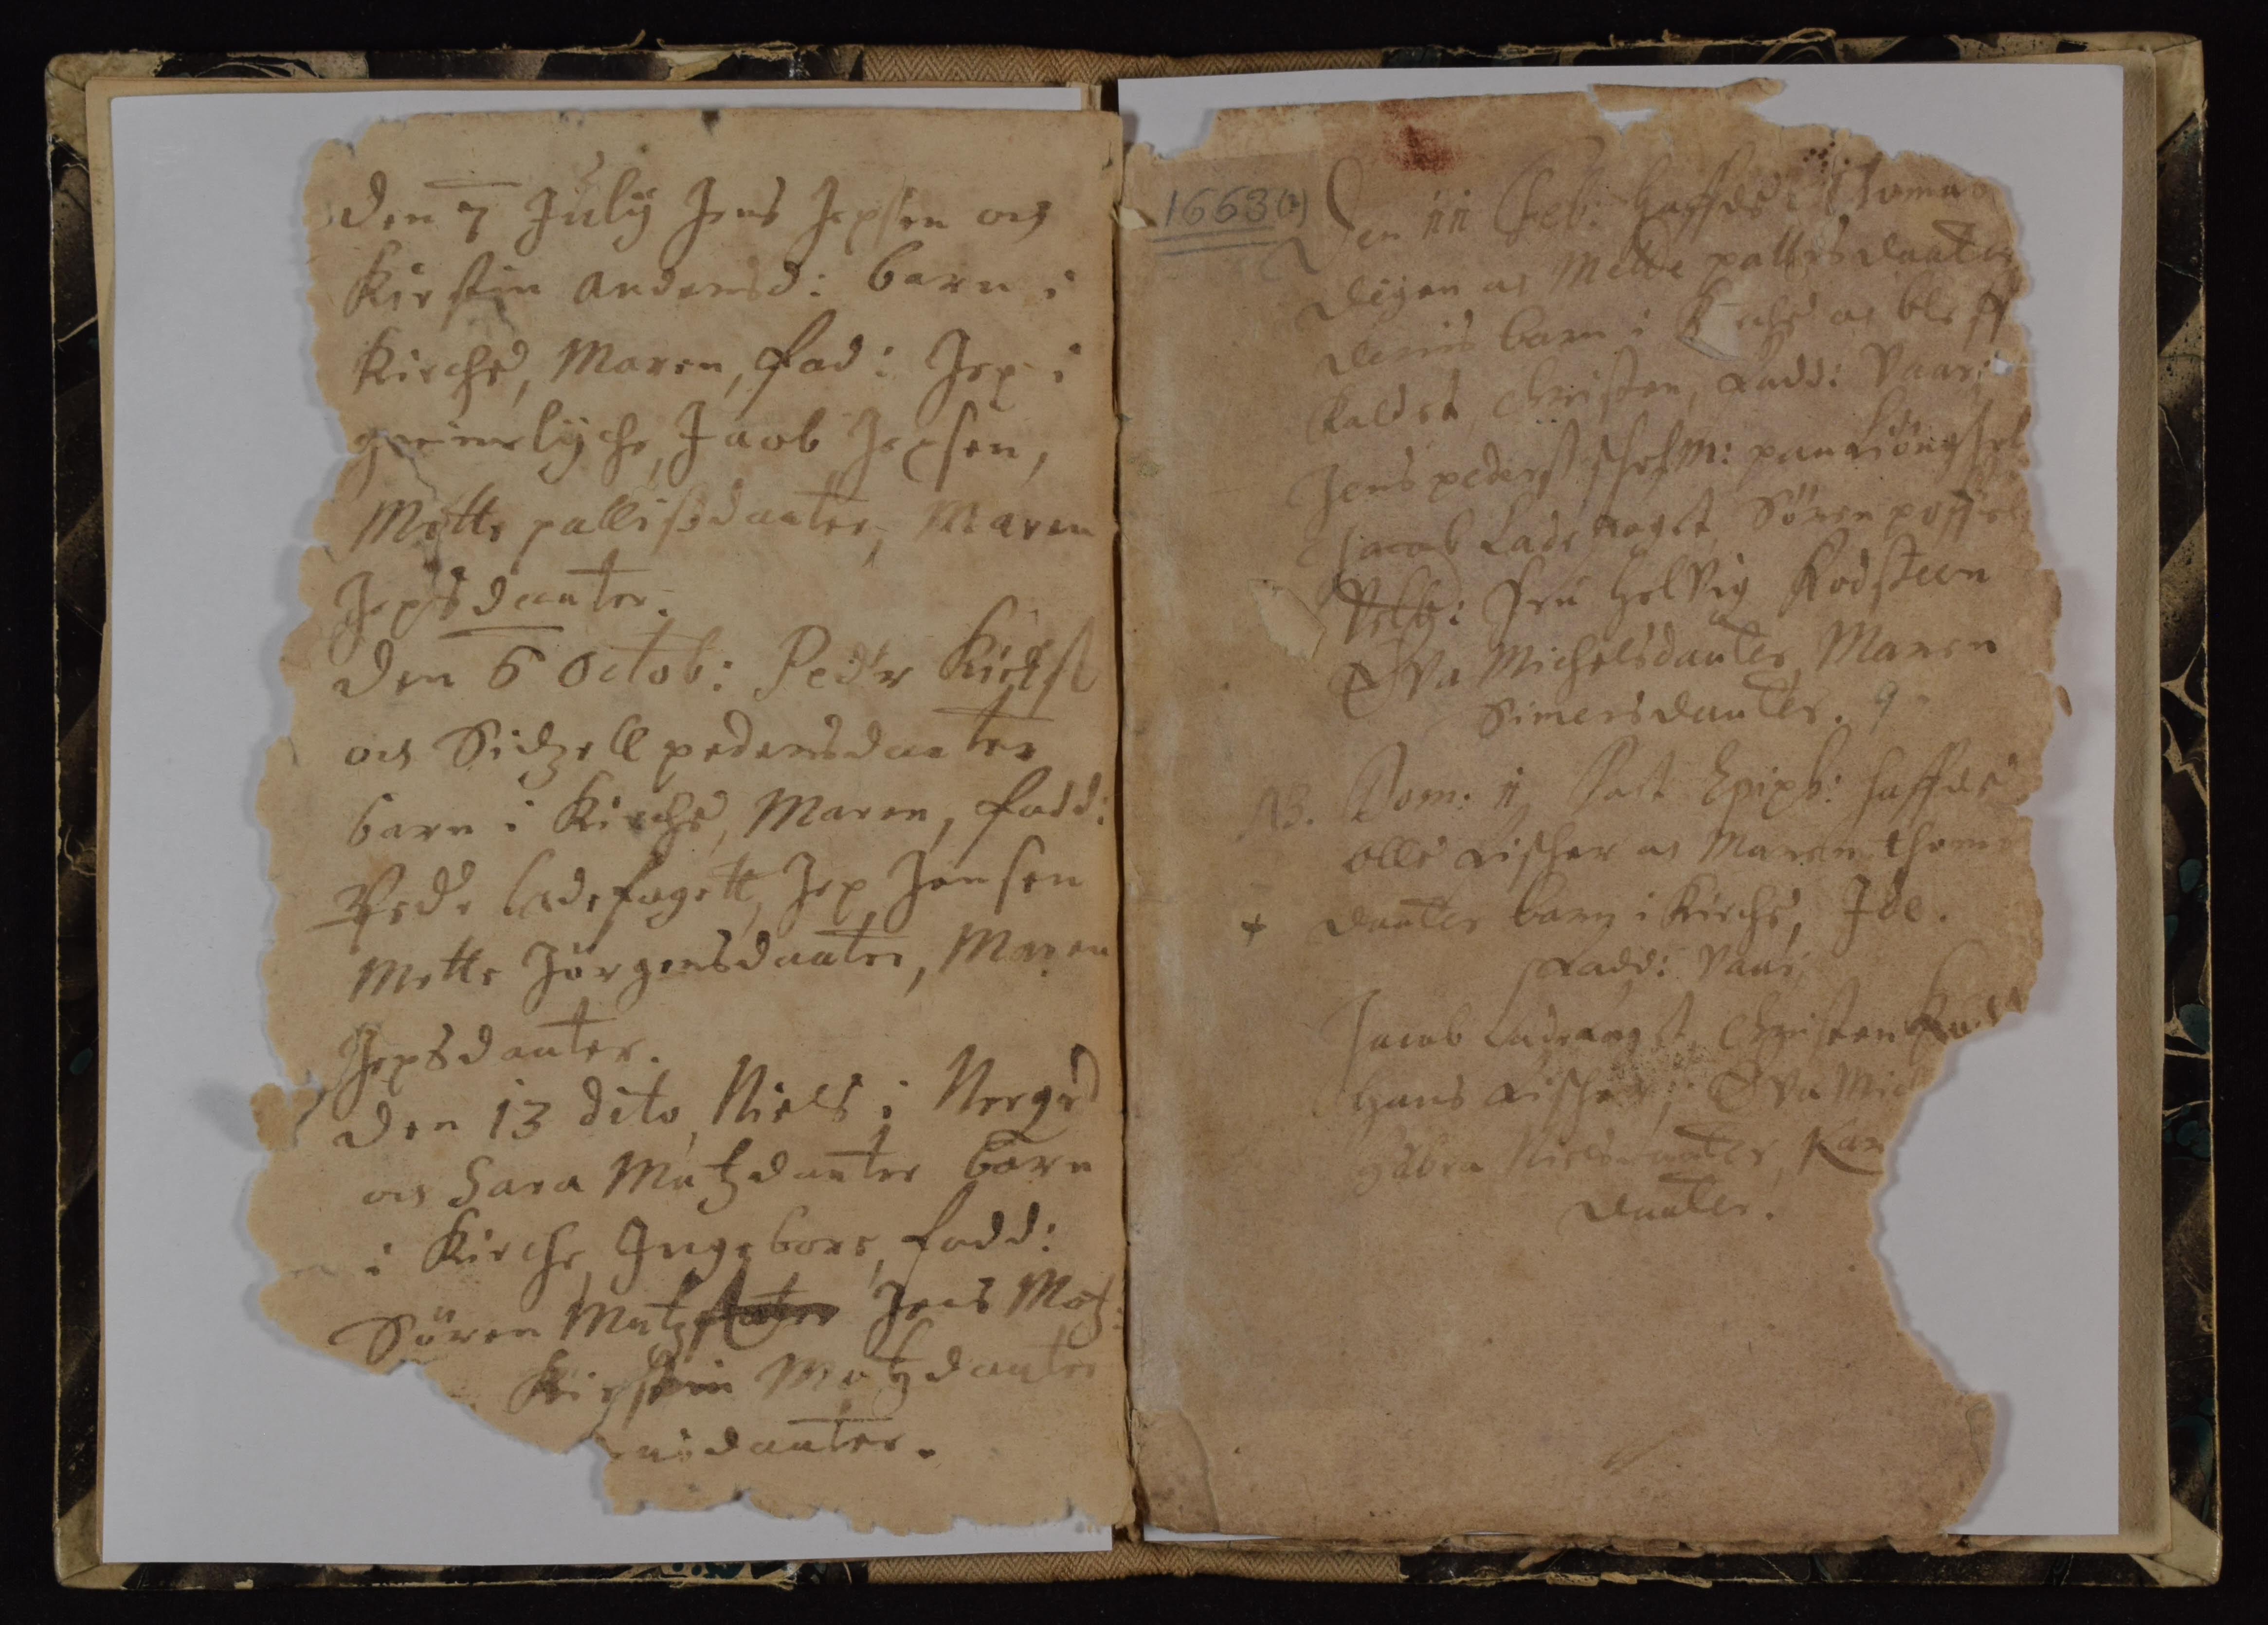Den 7 Julij Jens Jepsen och
Kirstin Andersd: barn i
Kirche, Maren, Fad: Jep i
Grimslyche, Jaob Jepsen,
Mette pallisdaater Maren
Jeps daater.
Den 6 Octob: Peder Kie##
och Sidzell Pedersdaater
barn i Kirche, Maren, Fadd:
Pedr Ladefogett, Jep Jensen
Mette Jørgensdaater, Maren
Jepsdauter.
den 13 dito Niels i Nergrd
och Sara Matzdaater barn
i Kirche Ingebors Fadd:
Søren Matz ater Jens Matz:
Kirstin Matzdauter
  ##sdauter
Den 11 Feb: haffde Thomas
digen ochf Mette pallesdaatter
deris barn i Kirche och bleff
kaldet Christen, Fadd: vaar;
Jens Peders Scholm: paa Liønghol
Jacob Ladefogrt Søren poffuells
Velb: Fru Helvig Rodsteen
Eva Michelsdauter Maren
Simensdautter.
NB. Dom. 11 Post Epiph: haffde
Olle Fisher och Maren Thoms
Dauter barn i Kirche, Ide.
Fadd: vaar;
Jacob Ladefoget Christen K##
Hans Fisher, Eva Mic
Babra Nielsdaater Kar
daater.
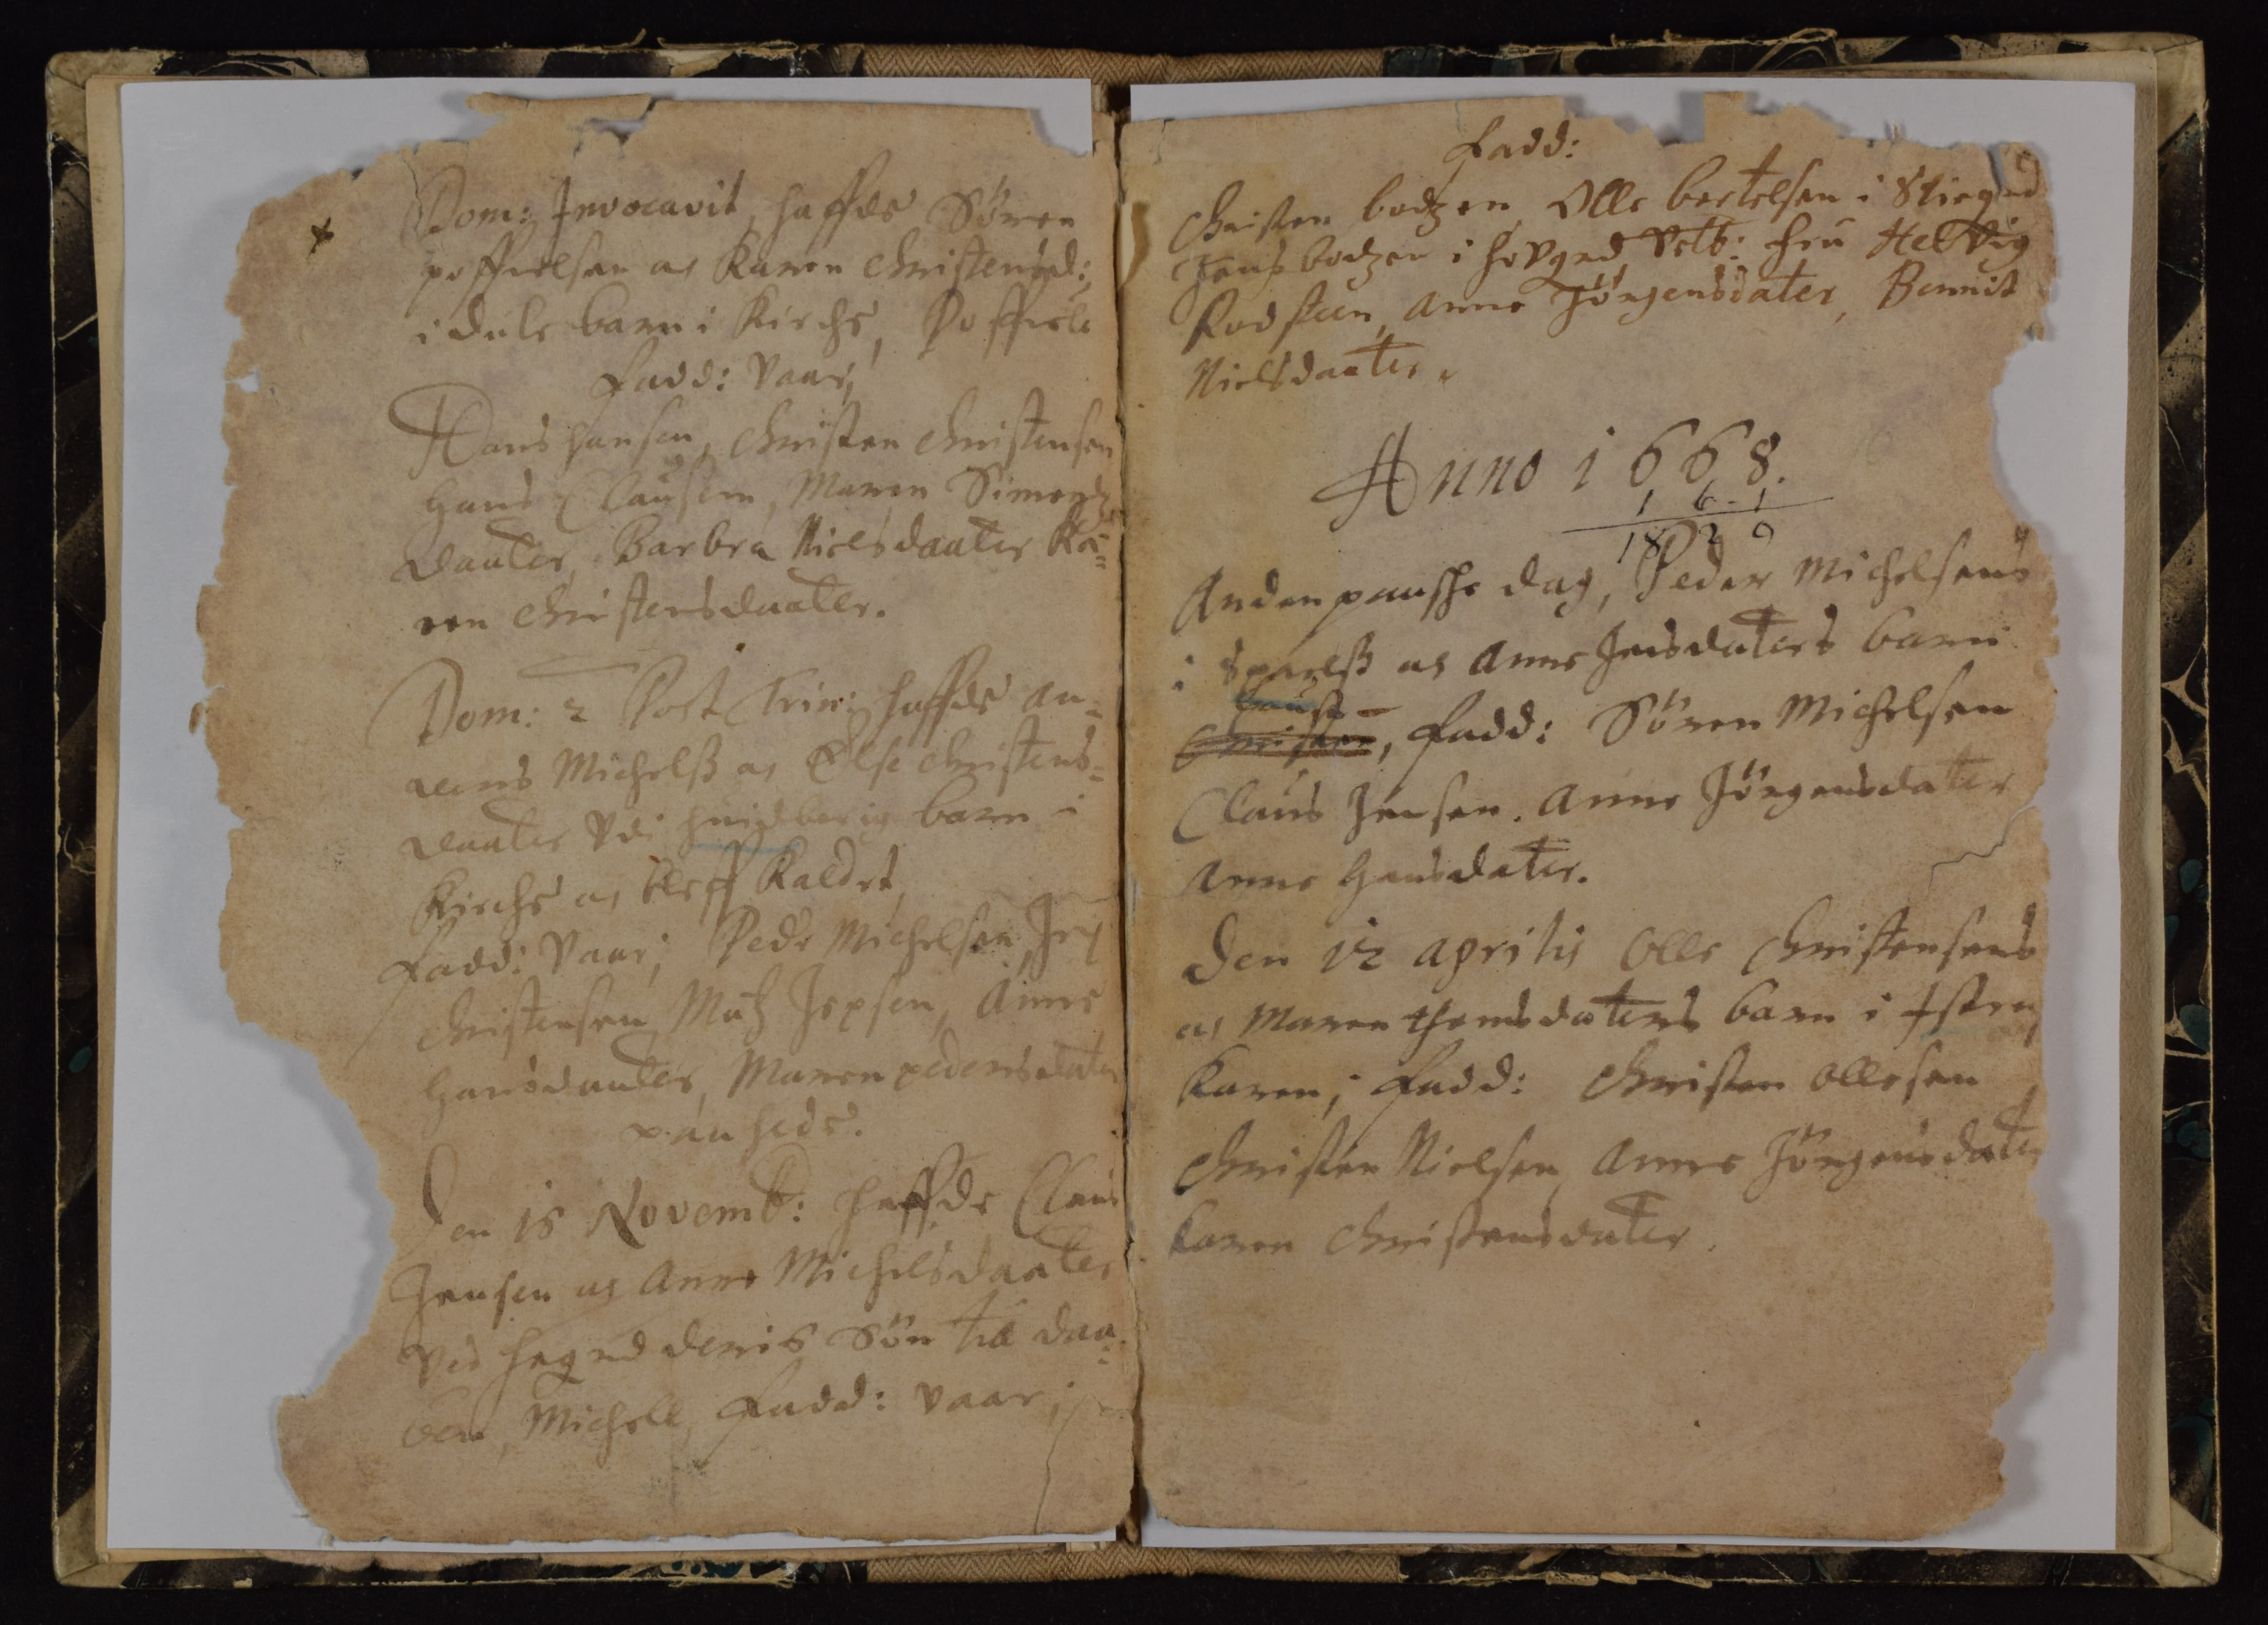Dom: Invocavit haffde Søren
poffuelsen och Karen Christensd:
i dule barn i Kirche Poffuell
Fadd: vaar,
Hans Hansen, Christen Christensen
Hans Clausen, Maren Simons
dauter, Barbra Nielsdaatter Ka¬
ren Christensdaater.
Dom: 2 Post Trinit: haffde An¬
ders Michelss og Else Christens¬
daater vdi Hvidberig barn i
Kirche och bleff kaldet,
Fadd: vaar; Pedr Michelson Jep
Christensen, Matz Jepsen, Anne
Hansdatter Maren pedersdater
paa heds.
Den 18 Novemb: haffde Claus
Jensen oc Anne Michelsdaater
ved Hegned deris Søn til Daa¬
ben Michell Fadd: Vaar;
Fadd:
Christen bodzen Olle bertelsen i Stiegrd
Hans Bodzen i hovgrd Velb: Fru Helvig
Rodsteen, Anne Jørgensdater Bennit
Nielsdaatter.
Anno 1668
161
1829
Anden panshe dag, Peder Michelsens
i Spaelss och Anne Jensdatters barn
Laust
Christen, Fadd. Søren Michelsen
Claus Jensen. Anne Jørgensdater
Anne Hansdater.
Den 12 aprilis Olle Christensens
och Maren Thomsdaters barn i Istrup
Karen; Fadd: Christen Ollesen
Christen Nielsen Anne Jørgensdater
Karen Christensdater